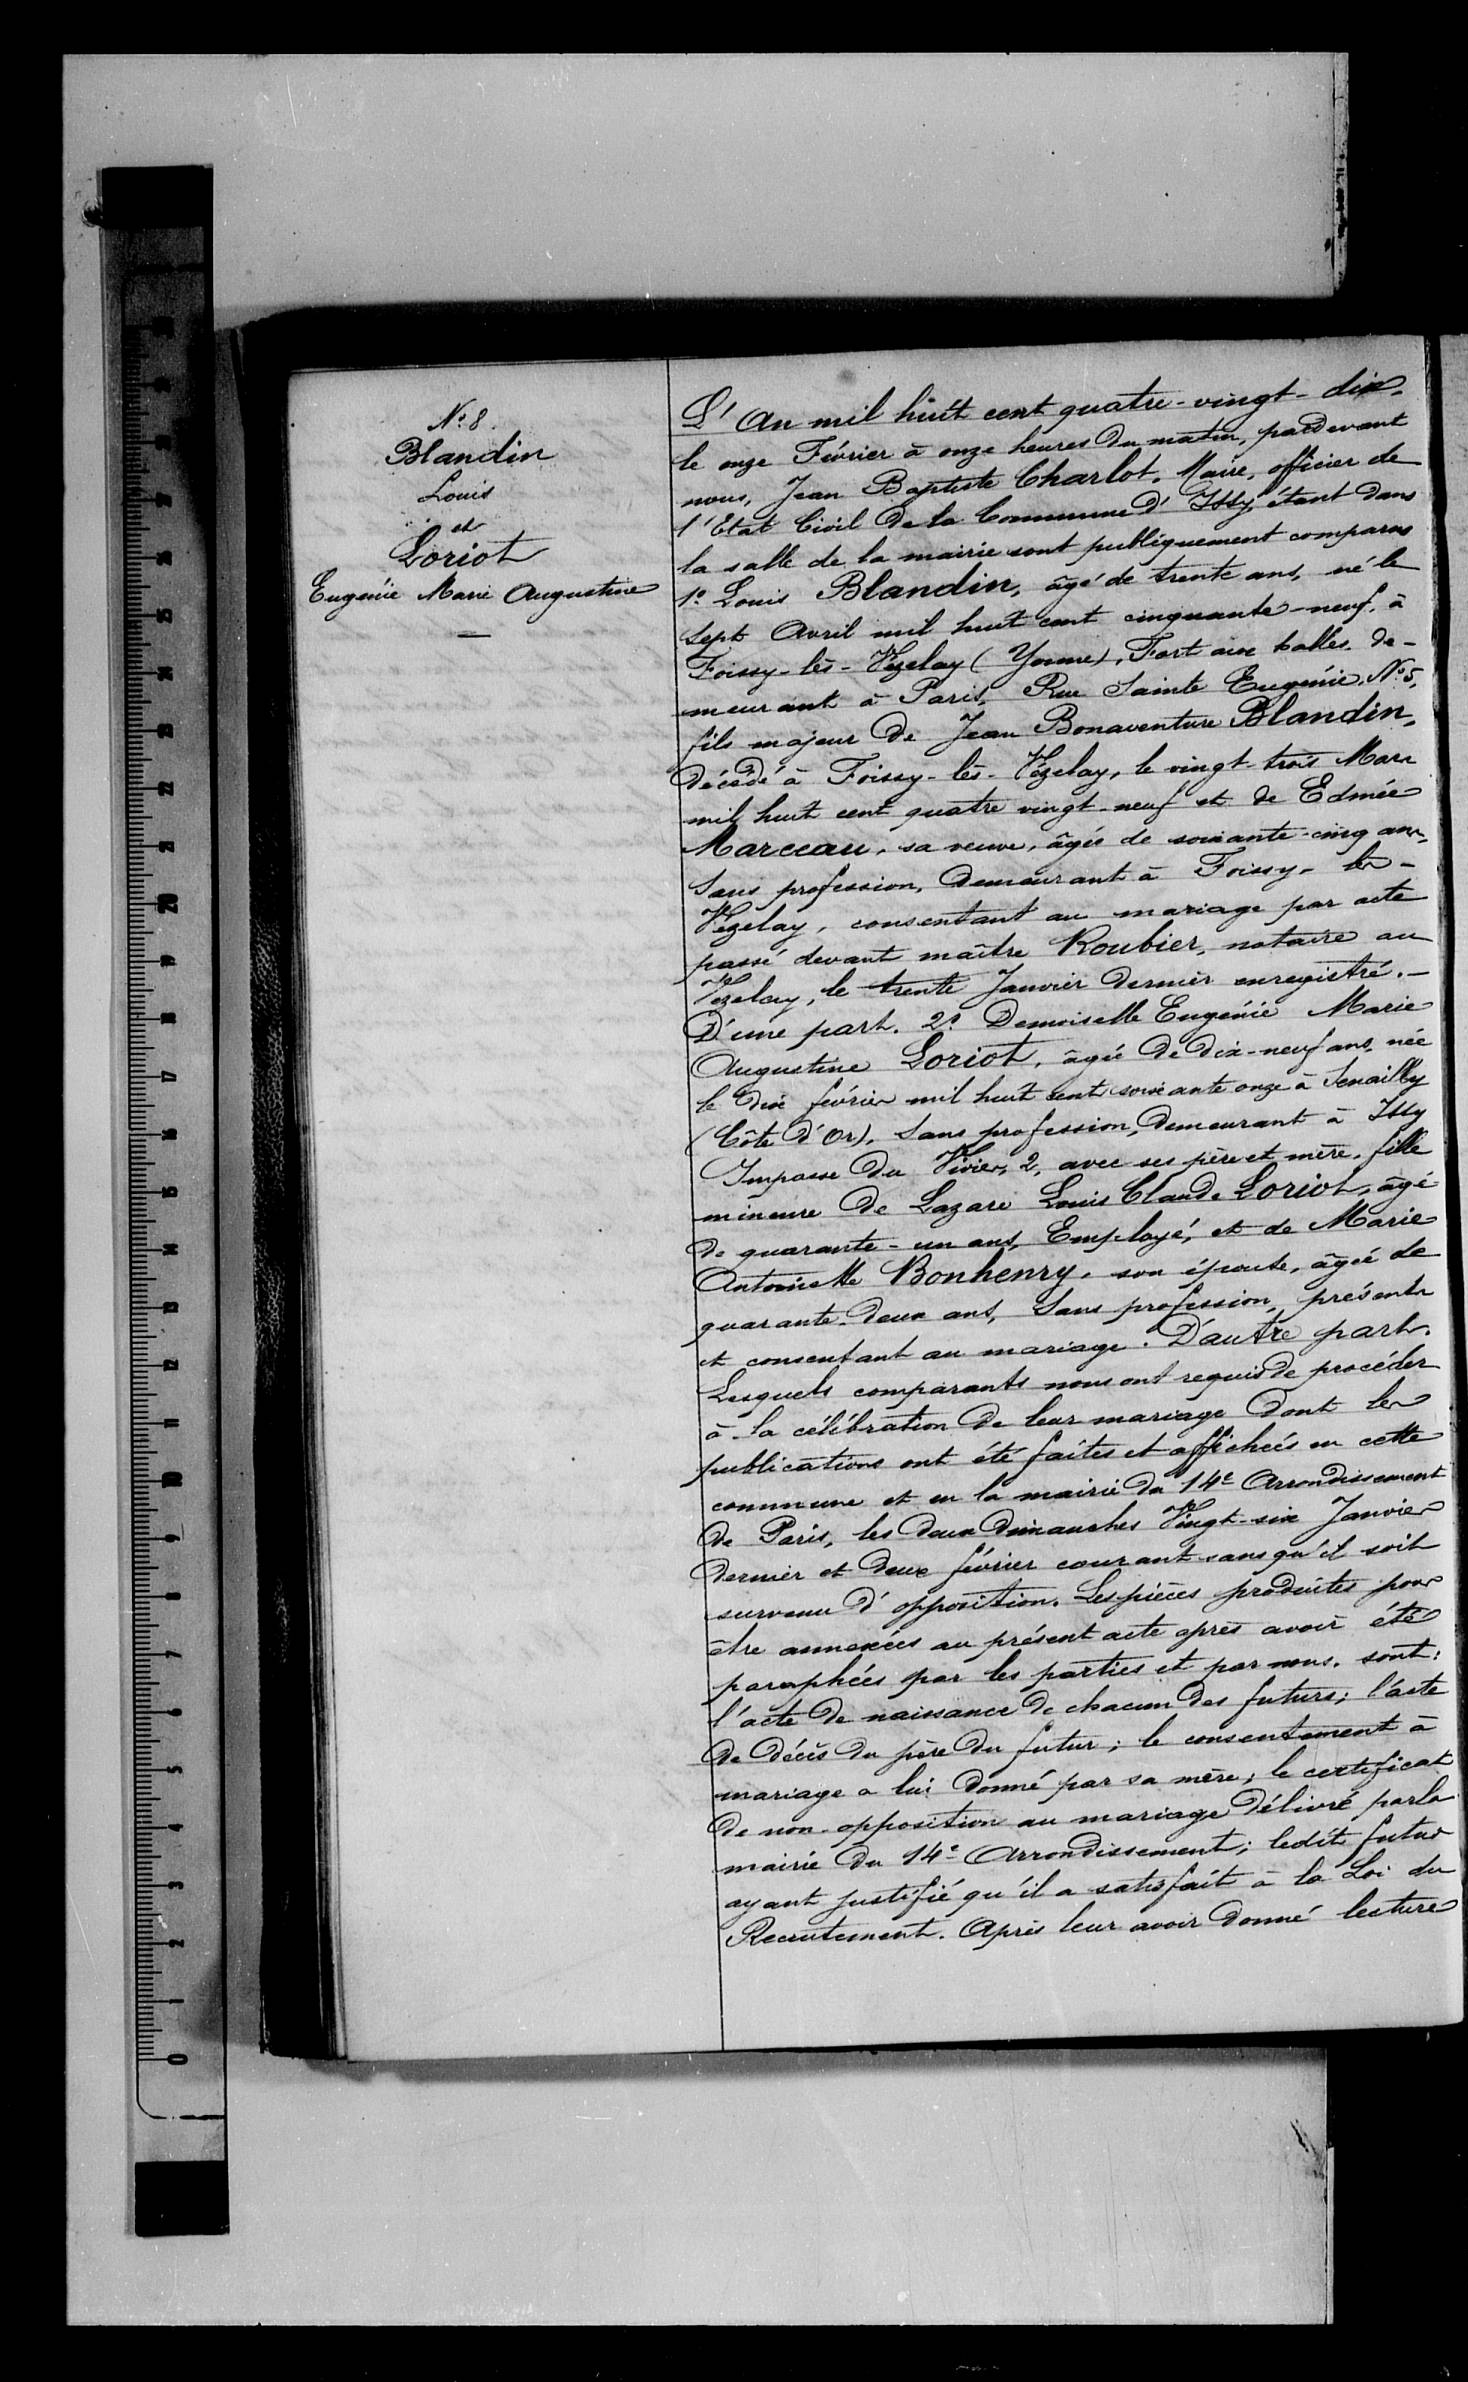N°8
Blandin
Louis
et
Loriot
Eugénie Marie Augustine
L'An mil huit cent quatre-vingt-dix
le onze Février à onze heures du matin, pardevant
nous, Jean Baptiste Charlot, Maire, officier de
l'Etat Civil de la Commune d'Issy, étant dans
la salle de la mairie sont publiquement comparu
1°: Louis Blandin, âgé de trente ans, né le
sept Avril mil huit cent cinquante neuf, à
Foissy-les-Vezelay (Yonne), Fontaine halles, de-
meurant à Paris, Rue Saint Eugénie. N°5
fis majeur de Jean Bonaventure Blandin,
décédé à Foissy-le-Vezelay, le vingt-trois Mars
mil huit cent quatre vingt-neuf et de Edmée
Marceau, sa veuve, âgée de soixante-cinq ans,
sans profession, demeurant à Foissy-le-
Vezelay, consentant au mariage par acte
passé devant maître Roubier, notaire au
Vezelay, le trente Janvier dernier enregistré.-
D'une part. 2. Demoiselle Eugénie Marie
Augustine Loriot, âgée de dix-neuf ans, née
le dix février mil huit cent soixante onze à Senailly
(Côte d'Or), sans profession, demeurant à Issy
Impasse du Vivier, 2, avec ses père et mère, fille
mineure de Lazari Louis Claude Loriot, âgé
de quarante-un an, Employé, et de Marie
Antoinette Bonhenry, son épouse, âgée de
quarante-deux ans, sans profession, présente
et consentant au mariage. D'autre part,
Lesquels comparant nous ont requis de procéder
à la célébration de leur mariage dont les
publications ont été faites et affranchies en cette
commune et en la mairie du 14° Arrondissement
de Paris, les deux dimanches Vingt-six Janvier
dernier et deux février courant sans qu'il soit
survenu d'opposition. Les pièces produites pour
être annexées au présent acte après avoir été
paraphées par les parties et par nous, sont:
l'acte de naissance de chacun des futurs; l'acte
de décès du père du futur; le consentement à
mariage à lui donné par sa mère, le certificat
de non-opposition au mariage délivré par la
mairie du 14° Arrondissement; ledit futur
ayant justifié qu'il a satisfait à la Loi du
Recrutement. Après leur avoir donné lecture
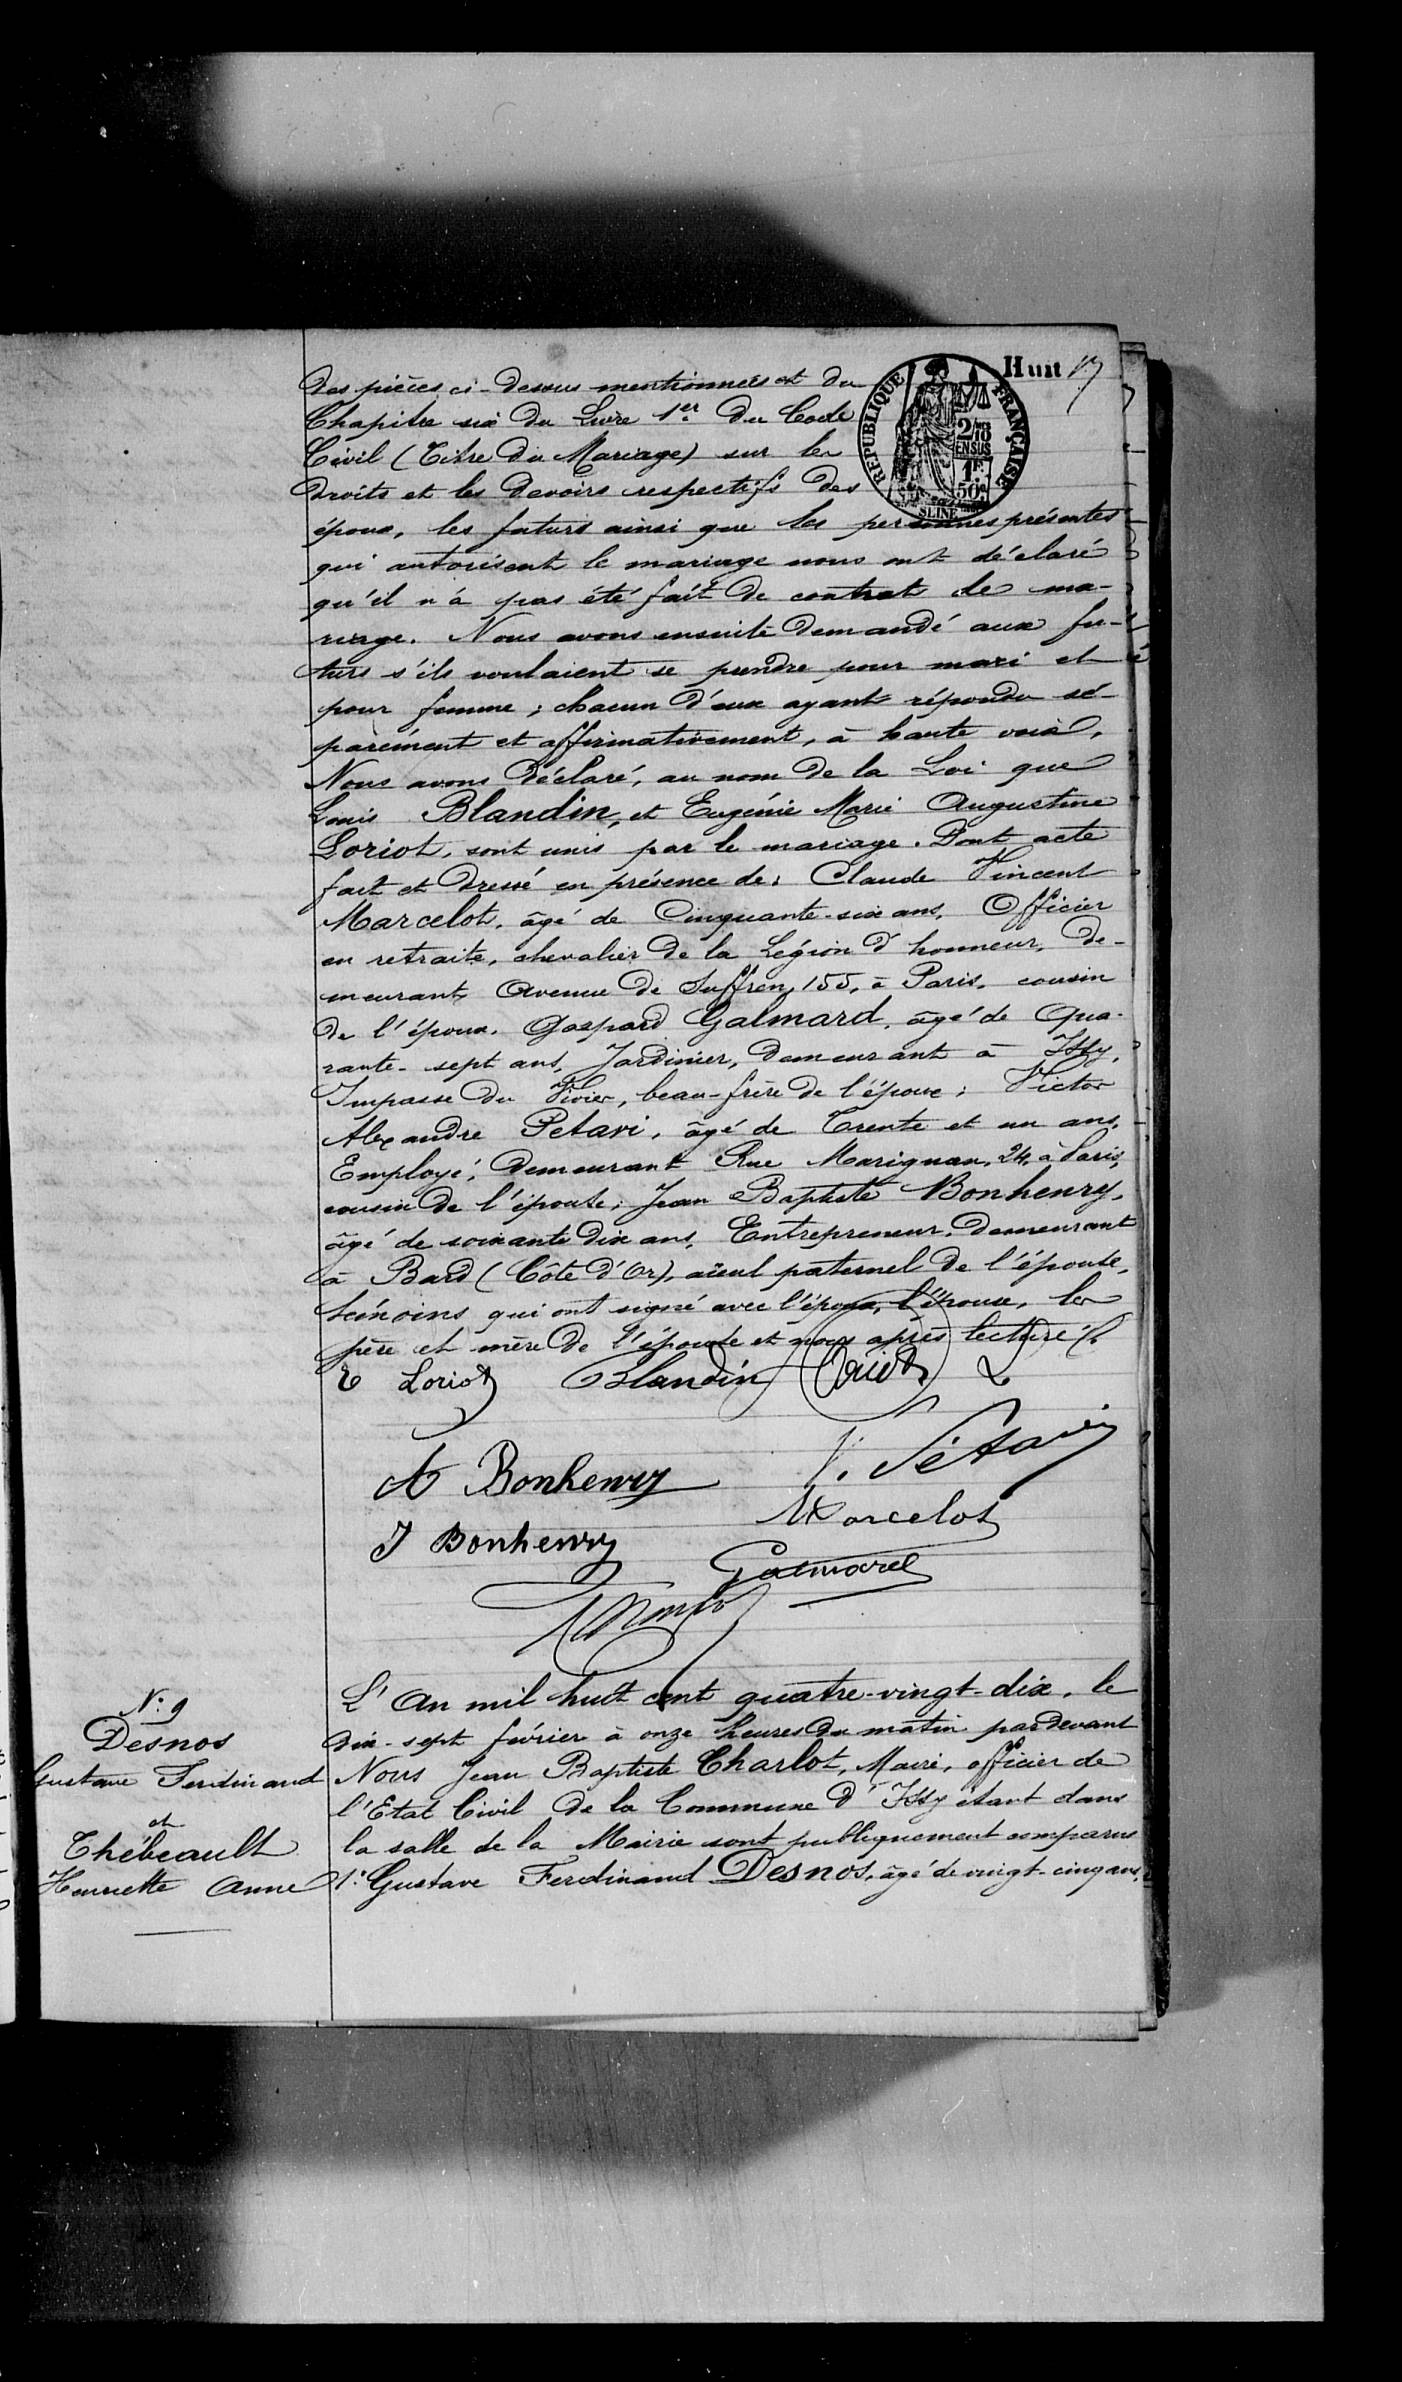des pièces ci-dessus mentionnées et du
Chapitre six du Livre 1er du Code
Civil (Titre du Mariage) sur les
droits et les devoirs respectifs des
époux, les futurs ainsi que les personnes présentes
qui autorisent le mariage nous ont déclaré
qu'il n'a pas été fait de contrat de ma-
riage. Nous avons aussitôt demandé aux fu-
turs s'ils voulaient se prendre pour mari et
pour femme, chacun d'eux ayant répondu sé
parément et affirmativement, à haute voix.
Nous avons déclaré, au nom de la Loi, que
Louis Blandin, et Eugénie Marie Augustine
Loriot, sont unis par le mariage. Dont acte
fait et dressé en présence de: Claude Vincent
Marcelot, âgé de cinquante-six ans, Officier
en retraite, chevalier de la Légion d'honneur, de-
meurant Avenue de Suffren, 155, à Paris, cousin
de l'époux. Gaspard Galmard, âgé de Qua-
rante-sept ans, Jardinier, demeurant à Issy
Impasse du Vivier, beau-frère de l'épouse: Victor
Alexandre Petavi, âgé de trente et un ans,
Employé: demeurant Rue Marignan, 24 à Paris,
cousin de l'épouse, Jean Baptiste Bonhenry,
âgé de soixante dix ans, Entrepreneur, demeurant
à Bard (Côte d'Or), aïeul paternel de l'épouse,
témoins qui ont signé avec l'épouse, l'époux, les
père et mère de l'épouse et nous après lecture.
N°9
Desnos
Gustave Ferdinand
et
Thébéault
Jeannette Anne
L'An mil huit cent quatre-vingt-dix, le
dix-sept février à onze heures du matin, pardevant
Nous Jean Baptiste Charlot, Maire, officier de
l'Etat Civil de la Commune d'Issy étant dans
la salle de la Mairie sont publiquement comparu
1° Gustave Ferdinand Desnos, âgé de vingt-cinq ans